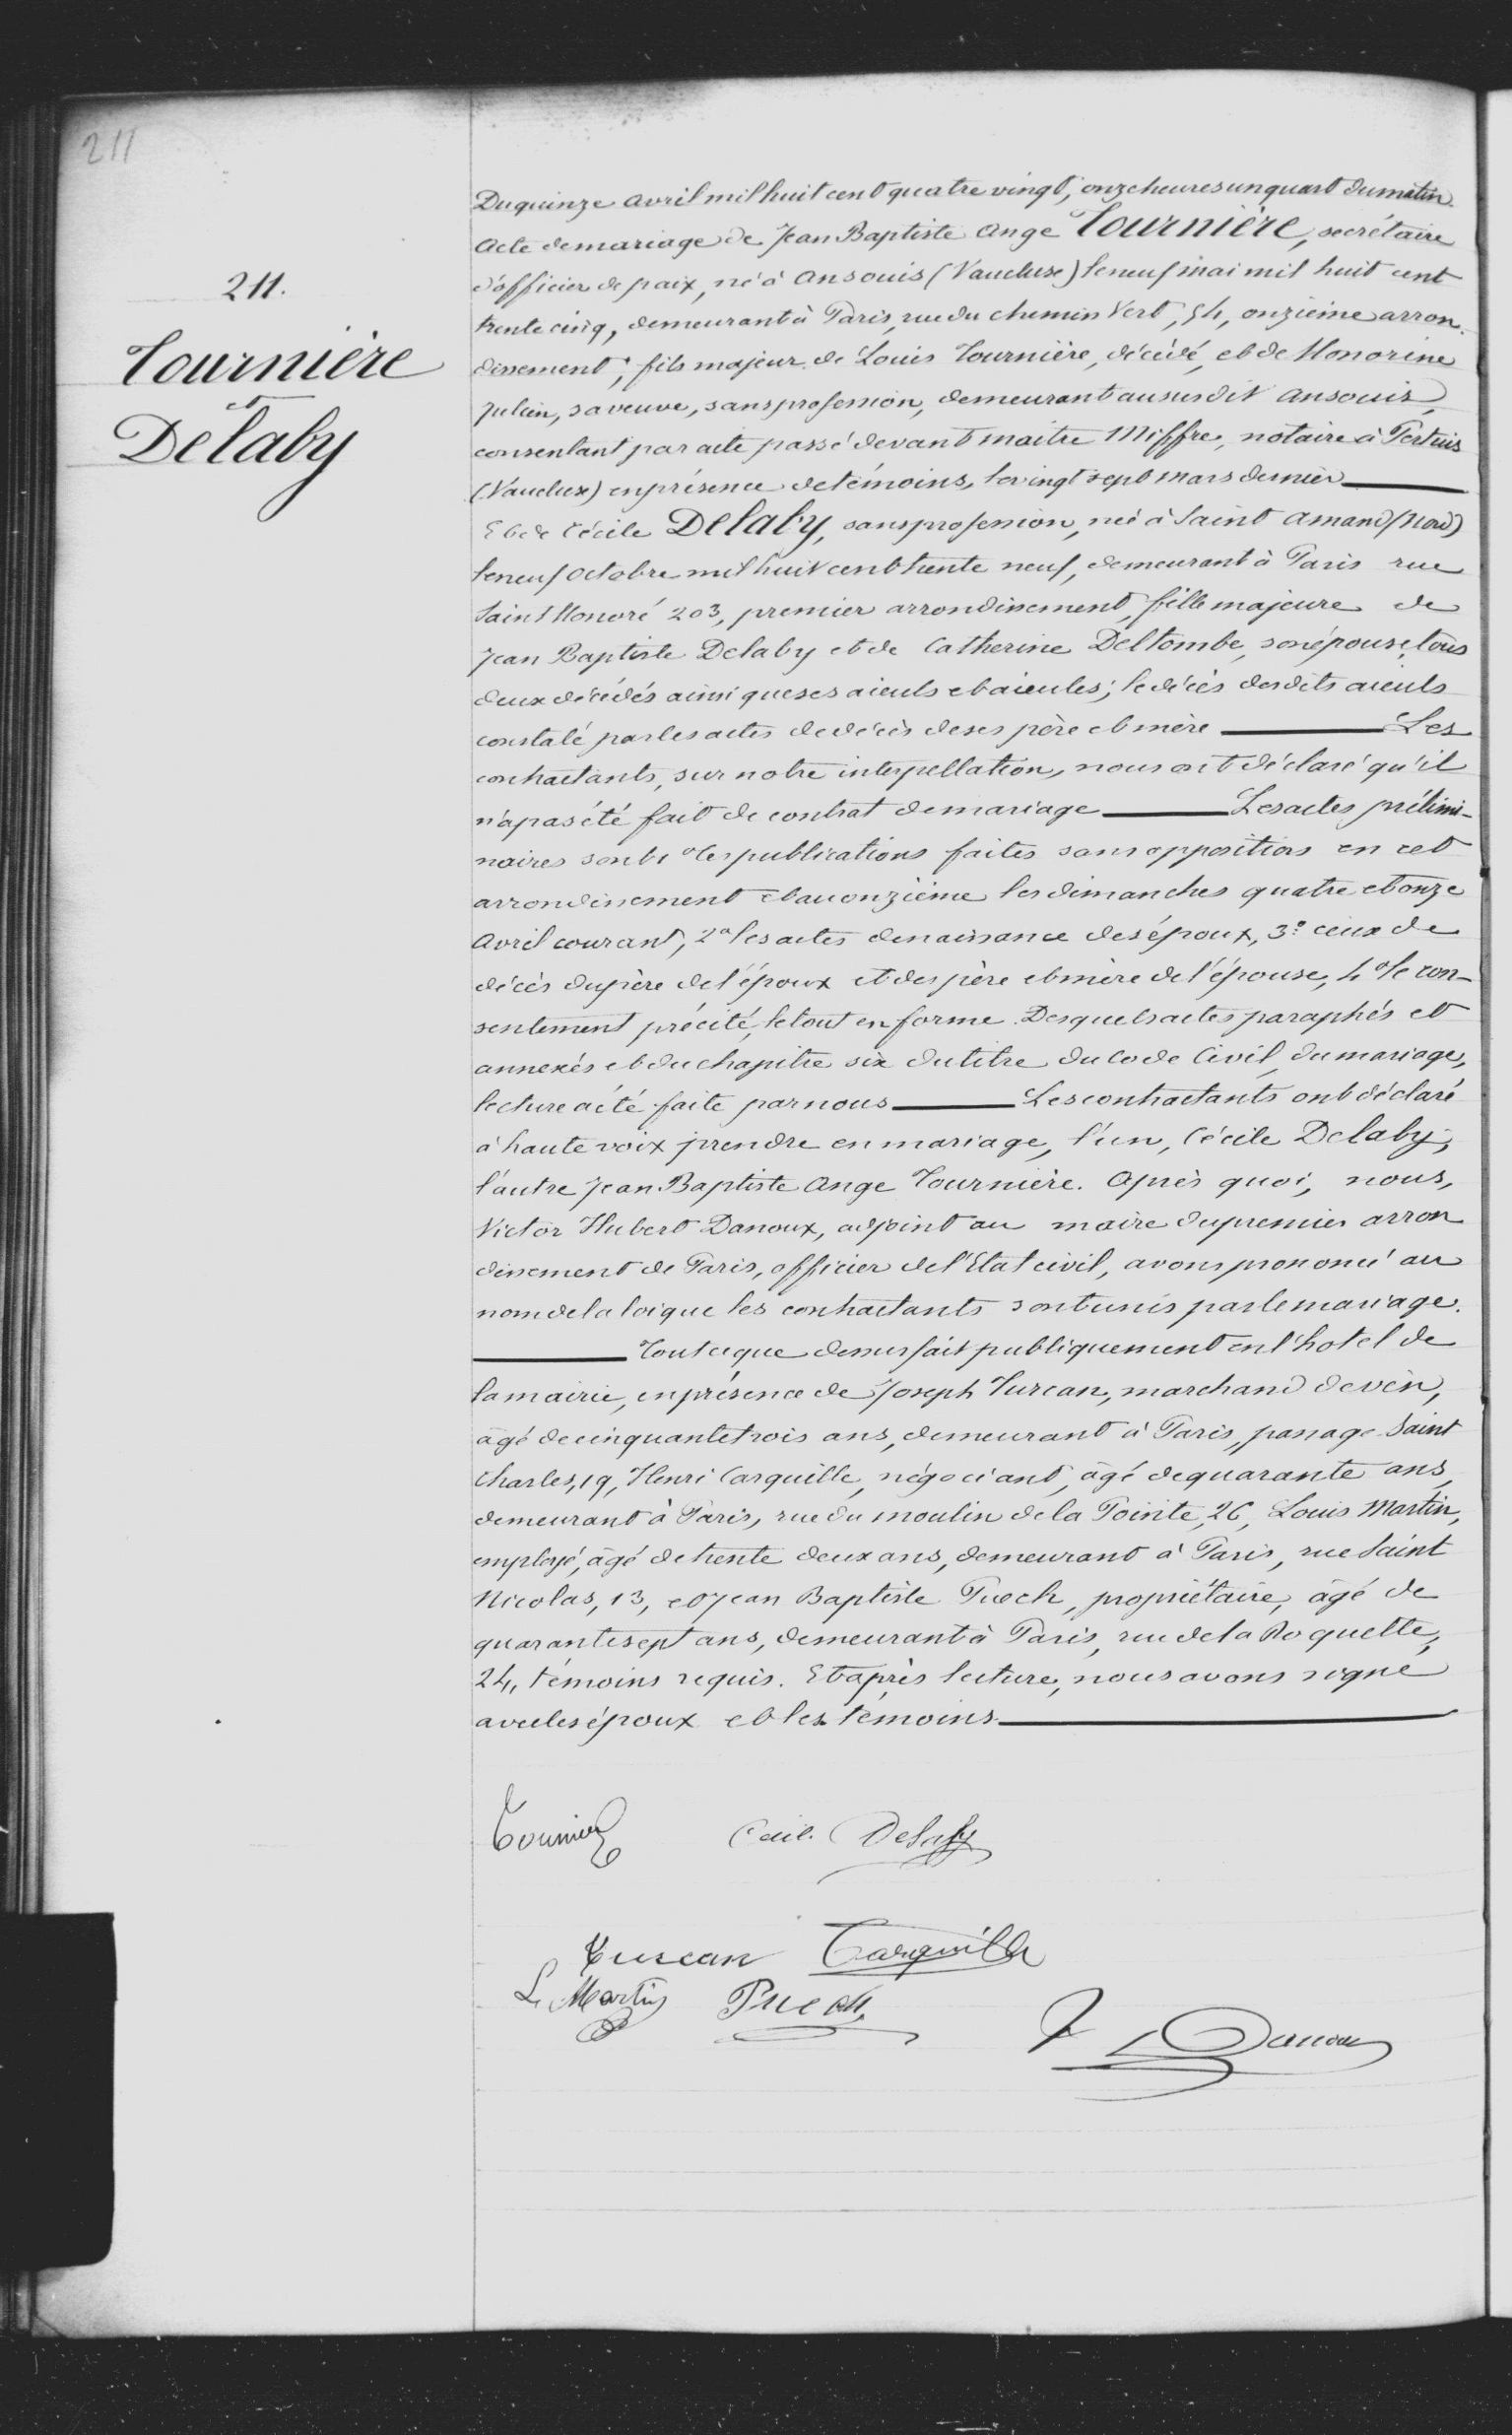211
Tournière
Delaby
Du quinze avril mil huit cent quatre vingt, onze heures un quart du martin
acte de mariage de Jean Baptiste Ange Tournière, secrétaire
d'officier de paix, né à Ansouis (Vaucluse) le neuf mai mil huit cent
trente cinq, demeurant à Paris, rue du Chemin Vert, 54, onzième arron
dissement; fils majeur de Louis Tournière, décédé, et de Honorine
Julien, sa veuve, sans profession, demeurant au sus dit ansouis
consentant par acte passé devant maitre Miffre, notaire à Pertuis
(Vaucluse) en présence de témoins, les cinq sept mars dernier-
Et de Cecil Delaby, sans profession, née à Saint Amand (Nord)
le neuf octobre mil huit cent trente neuf, demeurant à Paris rue
Saint Honoré 203, premier arrondissement fille majeur de
Jean Baptiste Delaby et de Catherine Deltombe, son épouse tous
deux décédés ainsi que ses aieuls et aieules; le décés des dit aieuls
constaté par les actes de décès de ses père et mère -Les
contractants, sur notre interpellation, nous ont déclaré qu'il
n'a pas été fait de contrat de mariage -Les actes prélimi-
naire sont les publications faites sans oppositions en cet
arrondissement et au onzième les dimanches quatre et onze
avril courant, 2° les actes de naissance des époux, 3° ceux de
décès du père de l'époux et des père et mère de l'épouse, 4° de con-
sentement précité le tout en forme desquels actes paraphés et
annexés et du chapitre six du titre du Code Civil du mariage
lecture a été faite par nous-Les contractants ont déclaré
à haute voix prendre en mariage, l'un, Cécile Delaby;
l'autre Jean baptiste Ange Tournière. Après quoi, nous,
Victor Hubert Danoux, adjoint au maire du premier arron
dissement de Paris, officier de l'Etat civil, avons prononcé au
nom de la loi que les contractants sont unis par le mariage.
-Tout ce que dessus fait publiquement en l'hotel de
la mairie, en présence de Joseph Turcan, marchand de vin,
âgé de cinquante trois ans, demeurant à Paris, passage Saint
Charles, 19, Henri Carquille, négociant, âgé de quarante ans,
demeurant à Paris, rue du moulin de la Pointe, 26, Louis Martin
employé, âgé de trente deux ans, demeurant à Paris, rue Saint
Nicolas, 13, Jean Baptiste Roch, propriétaire, âgé de
quarante sept ans, demeurant à Paris, rue de la Roquette,
24, témoins requis. Et après lecture, nous avons signé
avec les époux et les témoins -
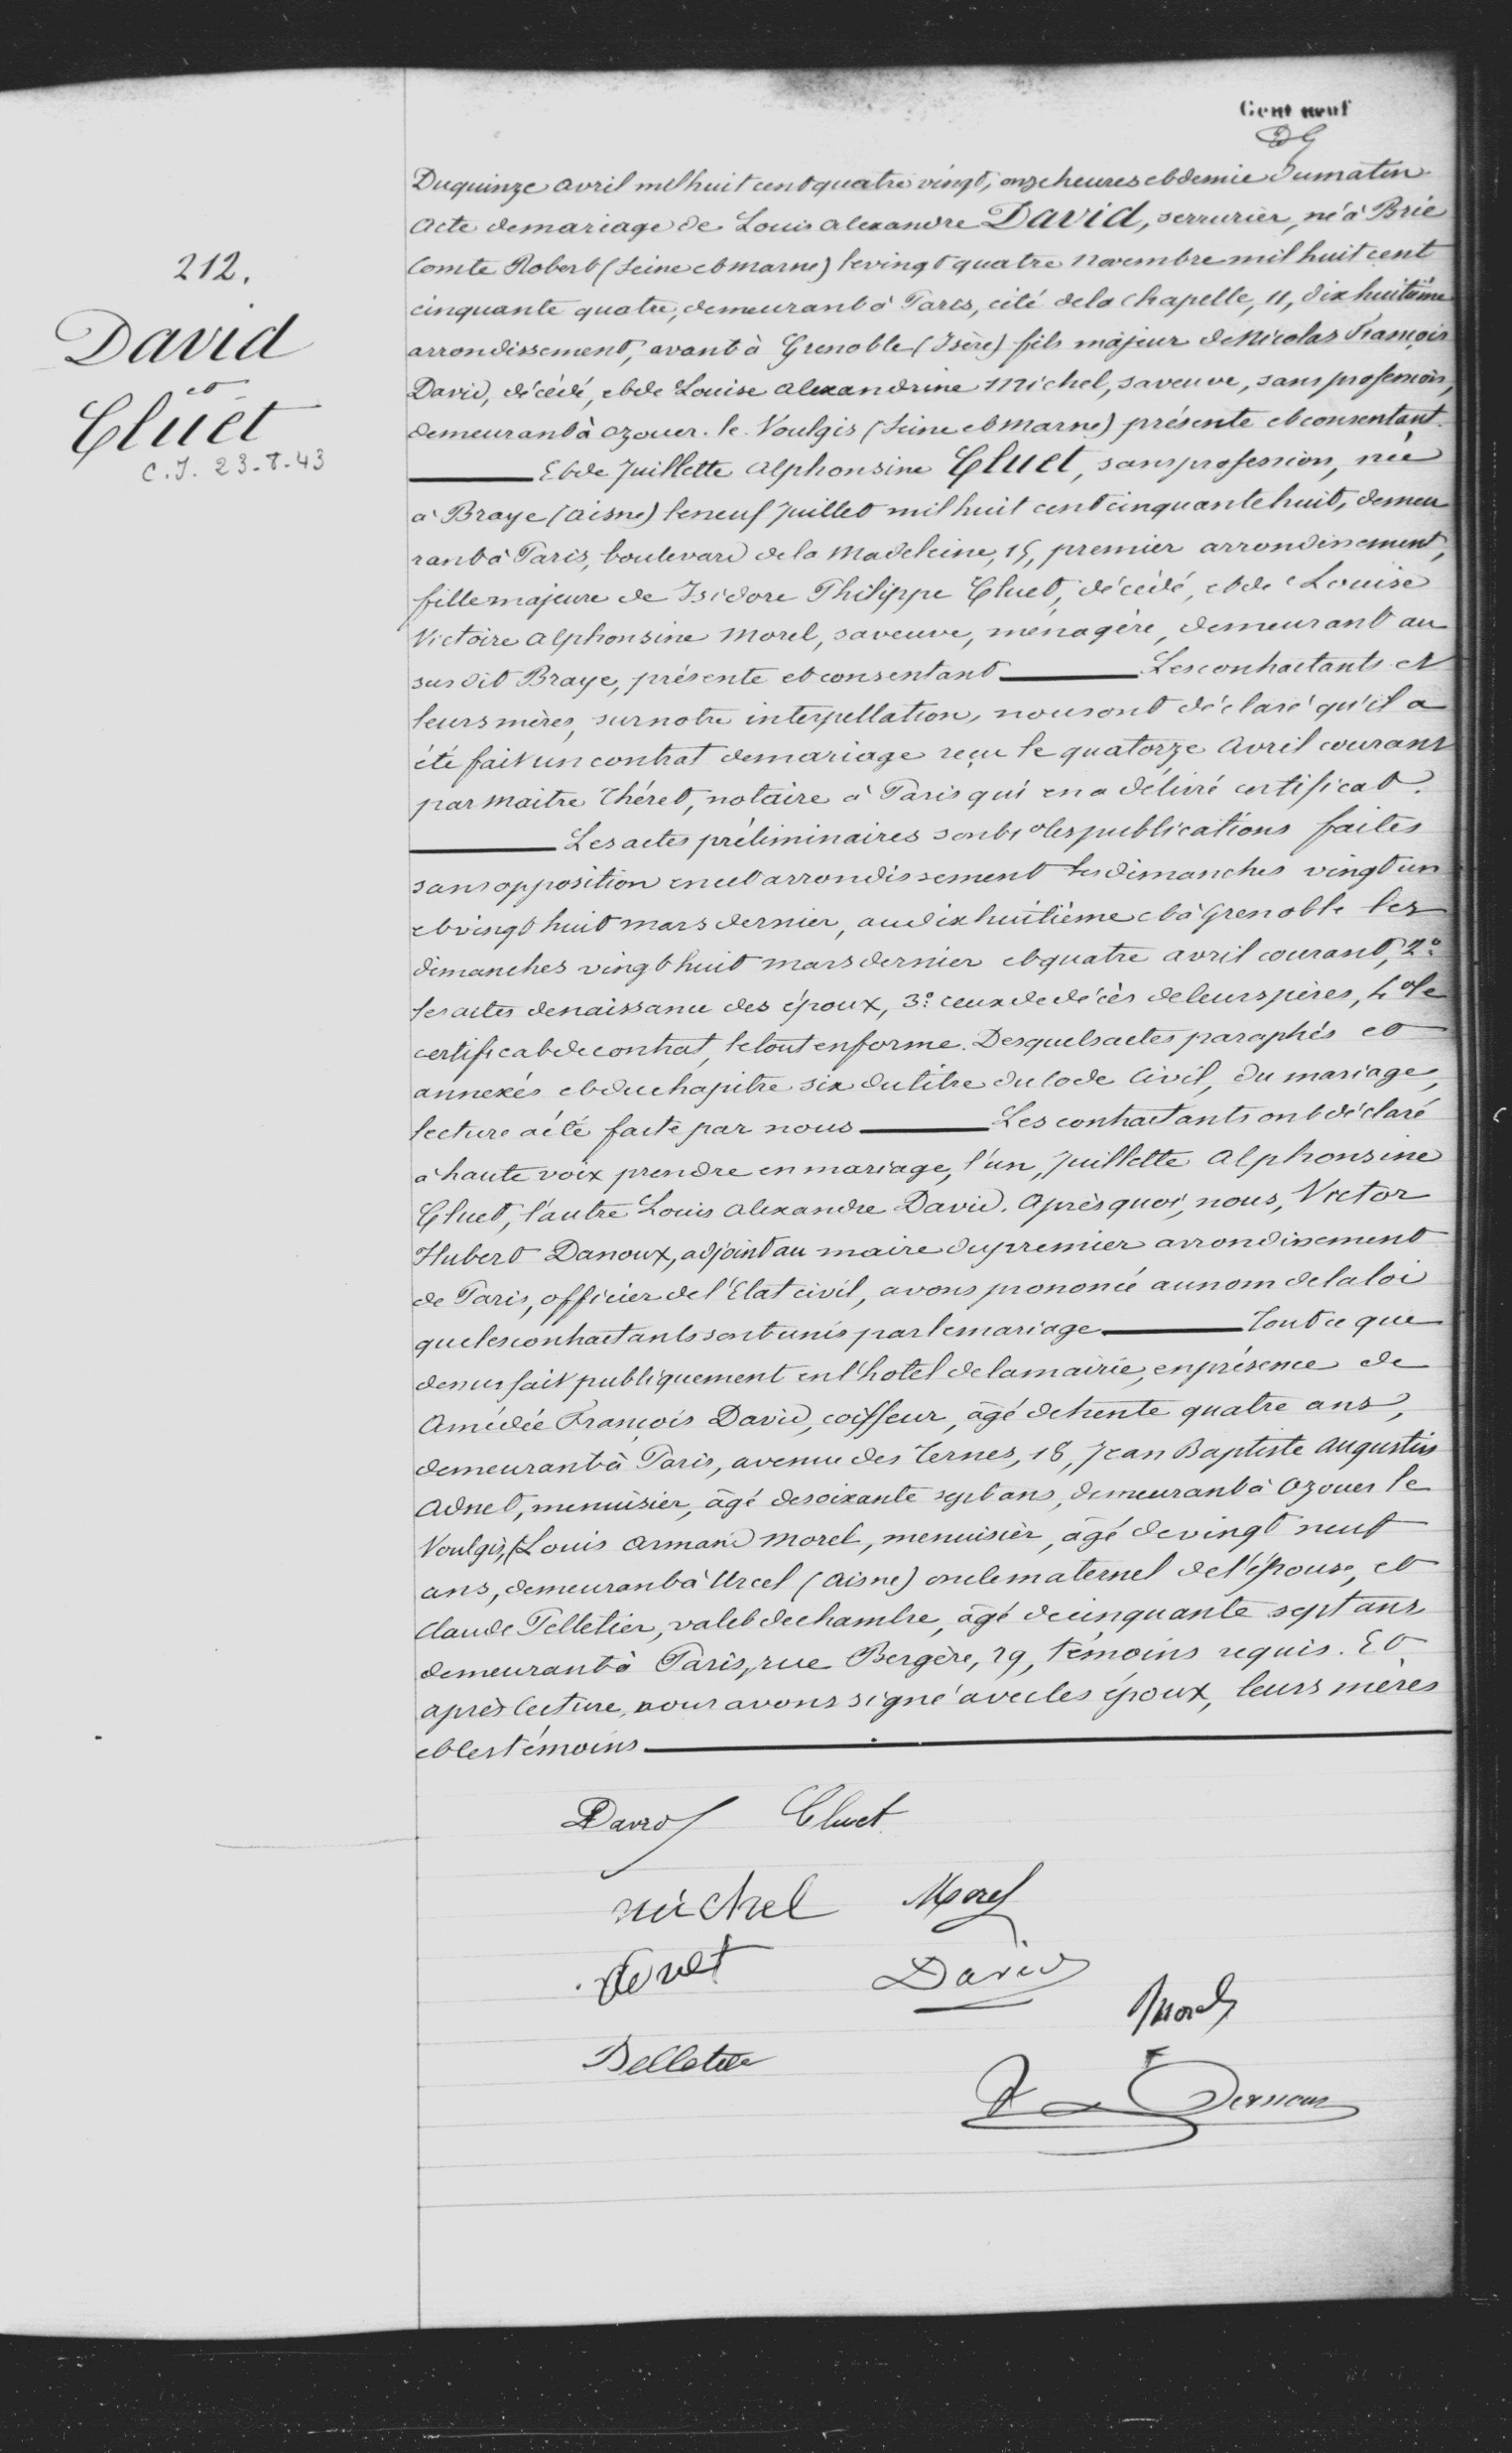212
David
et
Cluét
C.J. 23-8-43
Du quinze avril mil huit cent quatre vingt, onze heures et demie du matin.
acte de mariage de Louis Alexandre David, serrurier, né à Brie
Comte Robert (Seine et marne) le vingt quatre novembre mil huit cent
cinquante quatre, demeurant à Paris, cité de la chapelle, 11, dix huitième
arrondissement, avant à Grenoble (Isère) fils majeur de Nicolas François
David, décédé, et de Louise Alexandrine Michel, sa veuve, sans profession,
demeurant à Azouer le Voulgis (Seine et Marne) présente et consentant.
-Et de Juillette Alphonsine Gluet, sans profession, née
à Braye (Aisne) le neuf Juillet mil huit cent cinquante huit, demeu
rant à Paris, boulevard de la Madeleine, 15, premier arrondissement,
fille majeure de Isidore Philippe Gluet, décédé, et de Louise
Victoire Alphonsine Morel, sa veuve, ménagère, demeurant au
susdit Braye, présente et consentant -Les contractants et
leurs mères, sur notre interpellation, nous ont déclaré qu'il a
été fait un contrat demariage reçu le quatorze Avril courant
par maitre Théret, notaire à Paris qui en a délivré certificat.
-Les actes préliminaires sont 1° les publications faites
sans opposition en cette arrondissement les dimanches vingt un
et vingt huit mars dernier, au dix huitième et à Grenoble les
dimanches vingt huit mars dernier et quatre avril courant, 2°
les actes de naissance des époux, 3° ceux de décès de leurs pères, 4° le
certificat de contrat, le tout en forme. Desquels actes paraphés et
annexés et du chapitre six du titre du code Civil, du mariage,
lecture a été faite par nous-Les contractants ont déclaré
à haute-voix prendre en mariage, l'un, Juillette Alphonsine
Gluet, l'autre Louis Alexandre David. Après quoi, nous, Victor
Hubert Danoux, adjoint au maire du premier arrondissement
de Paris, officier de l'Etat civil, avons prononcé au nom de la loi
que les contractants sont unis par le mariage -Tout ce que
dans ces faits publiquement en l'hotel de la mairie, en présence de
Amédée François David, coiffeur, âgé de trente quatre ans,
demeurant à Paris, avenue des Ternes, 18, Jean Baptiste Augustin
Adnet, menuisier, âgé de soixante sept ans, demeurant à Azoues le
Voulgis, Louis Armani Morel, menuisier, âgé de vingt neuf
ans, demeurant à Urcel (Aisne) oncle maternel de l'épouse, et
Claude Pelletier, valet de chambre, âgé de cinquante sept ans
demeurant à Paris, rue Bergère, 29, témoins requis. Et
après lecture, nous avons signé avec les époux, leurs mères
et les témoins.